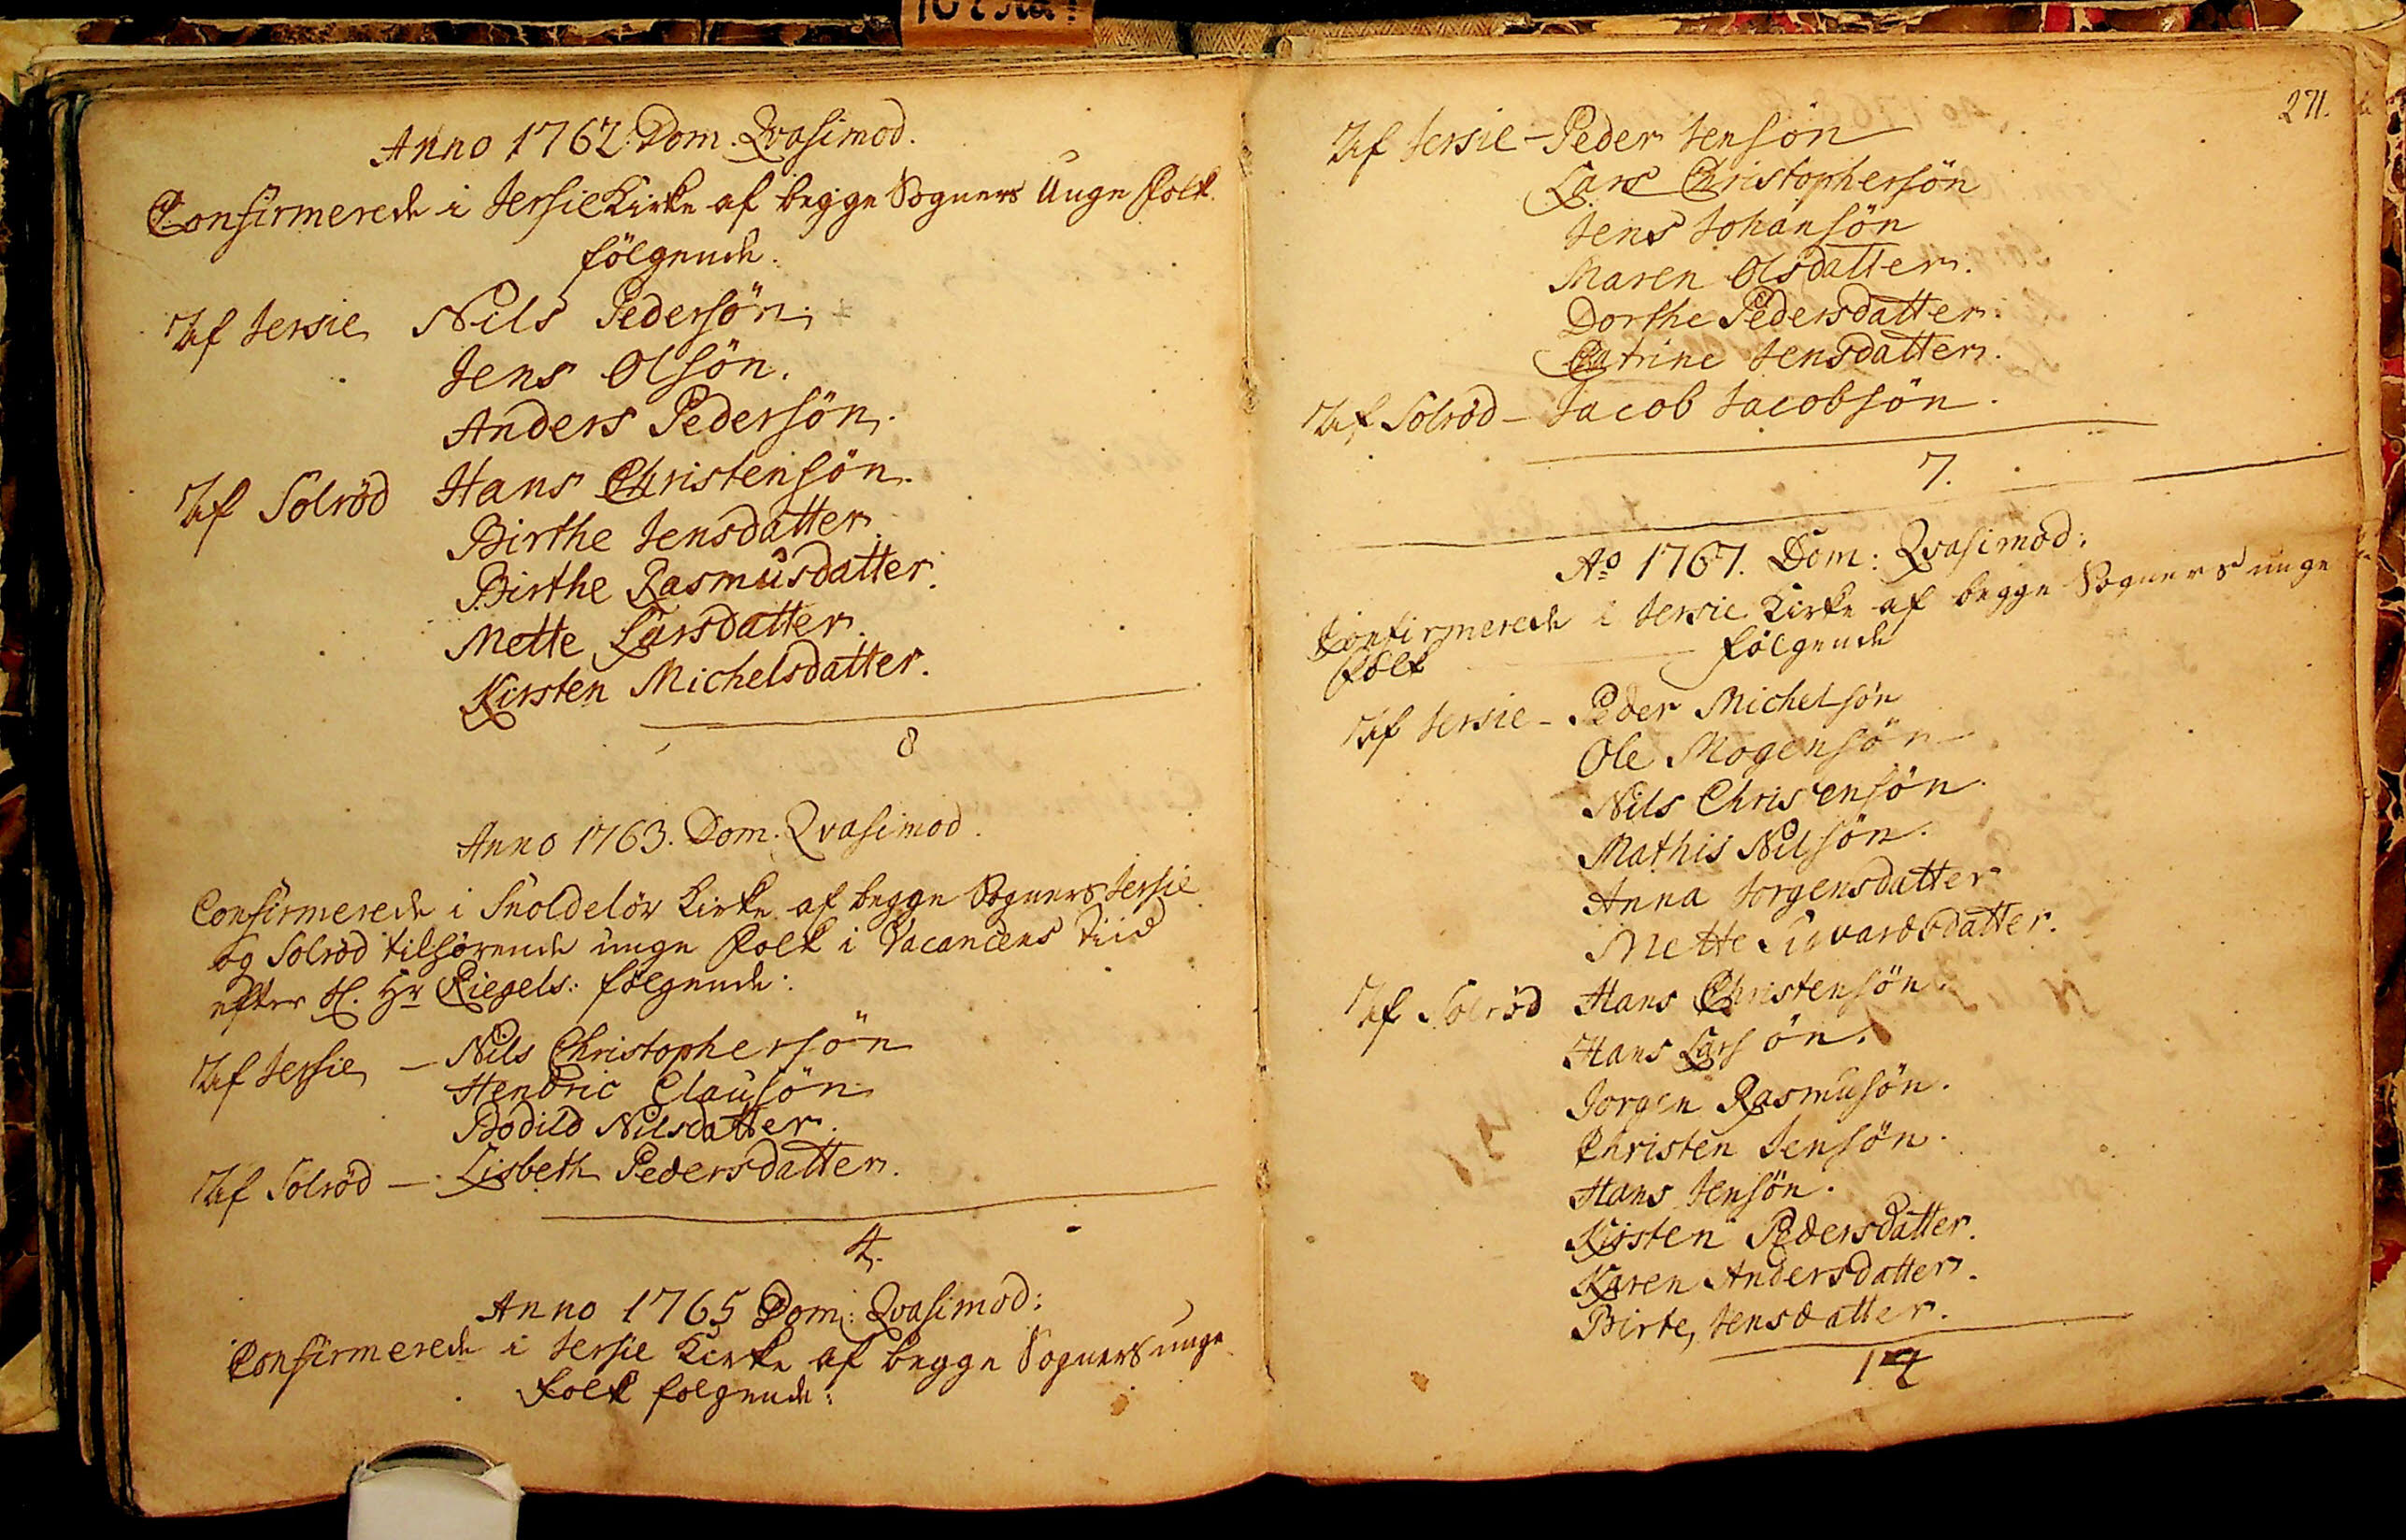Anno 1762. Dom. Qvasimod.
Confirmerede i Jersie Kirke af begge Sogners Unge Folk
følgende:
Af Jersie Nils Pedersøn.
Jens Olsøn.
Anders Pedersøn.
Af Solrød Hans Christensøn.
Birthe Jensdatter.
Birthe Rasmusdatter.
Mette Larsdatter.
Kirsten Michelsdatter.
8.
Anno 1763. Dom. Qvasimod.
Confirmerede i Snoldeløv Kirke af begge Sogners Jersie
og Solrød tilhørende unge Folk i Vacancens Tiid
efter Sl. Hr Riegels: følgende:
Af Jersie - Nils Christophersøn
Hendric Clausøn
Bodild Nilsdatter.
Af Solrød - Lisbeth Pedersdatter.
4.
Ano 1765 Dom: Qvasimod:
Confirmerede i Jersie Kirke af begge Sognes unge
folk følgende:
271.
Af Jersie - Peder Jensøn
Lars Christophersøn
Jens Johansøn
Maren Olsdatter.
Dorthe Pedersdatter.
Catrine Jensdatter.
Af Solrød - Jacob Jacobsøn.
7.
Ao 1767. Dom: Qvasimod:
Confirmerede i Jersie Kirke af begge Sogners unge
Folk
følgende
Af Jersie - Peder Michelsøn
Ole Mogensøn
Nils Christensøn
Mathis Nilsøn.
Anna Jørgensdatter
Mette Sigvardsdatter.
Af Solrød Hans Christensøn.
Hans Larsøn.
Jørgen Rasmusøn.
Christen Jensøn.
Hans Jensøn.
Kirsten Pedersdatter.
Karen Andersdatter.
Birte Jensdatter.
14
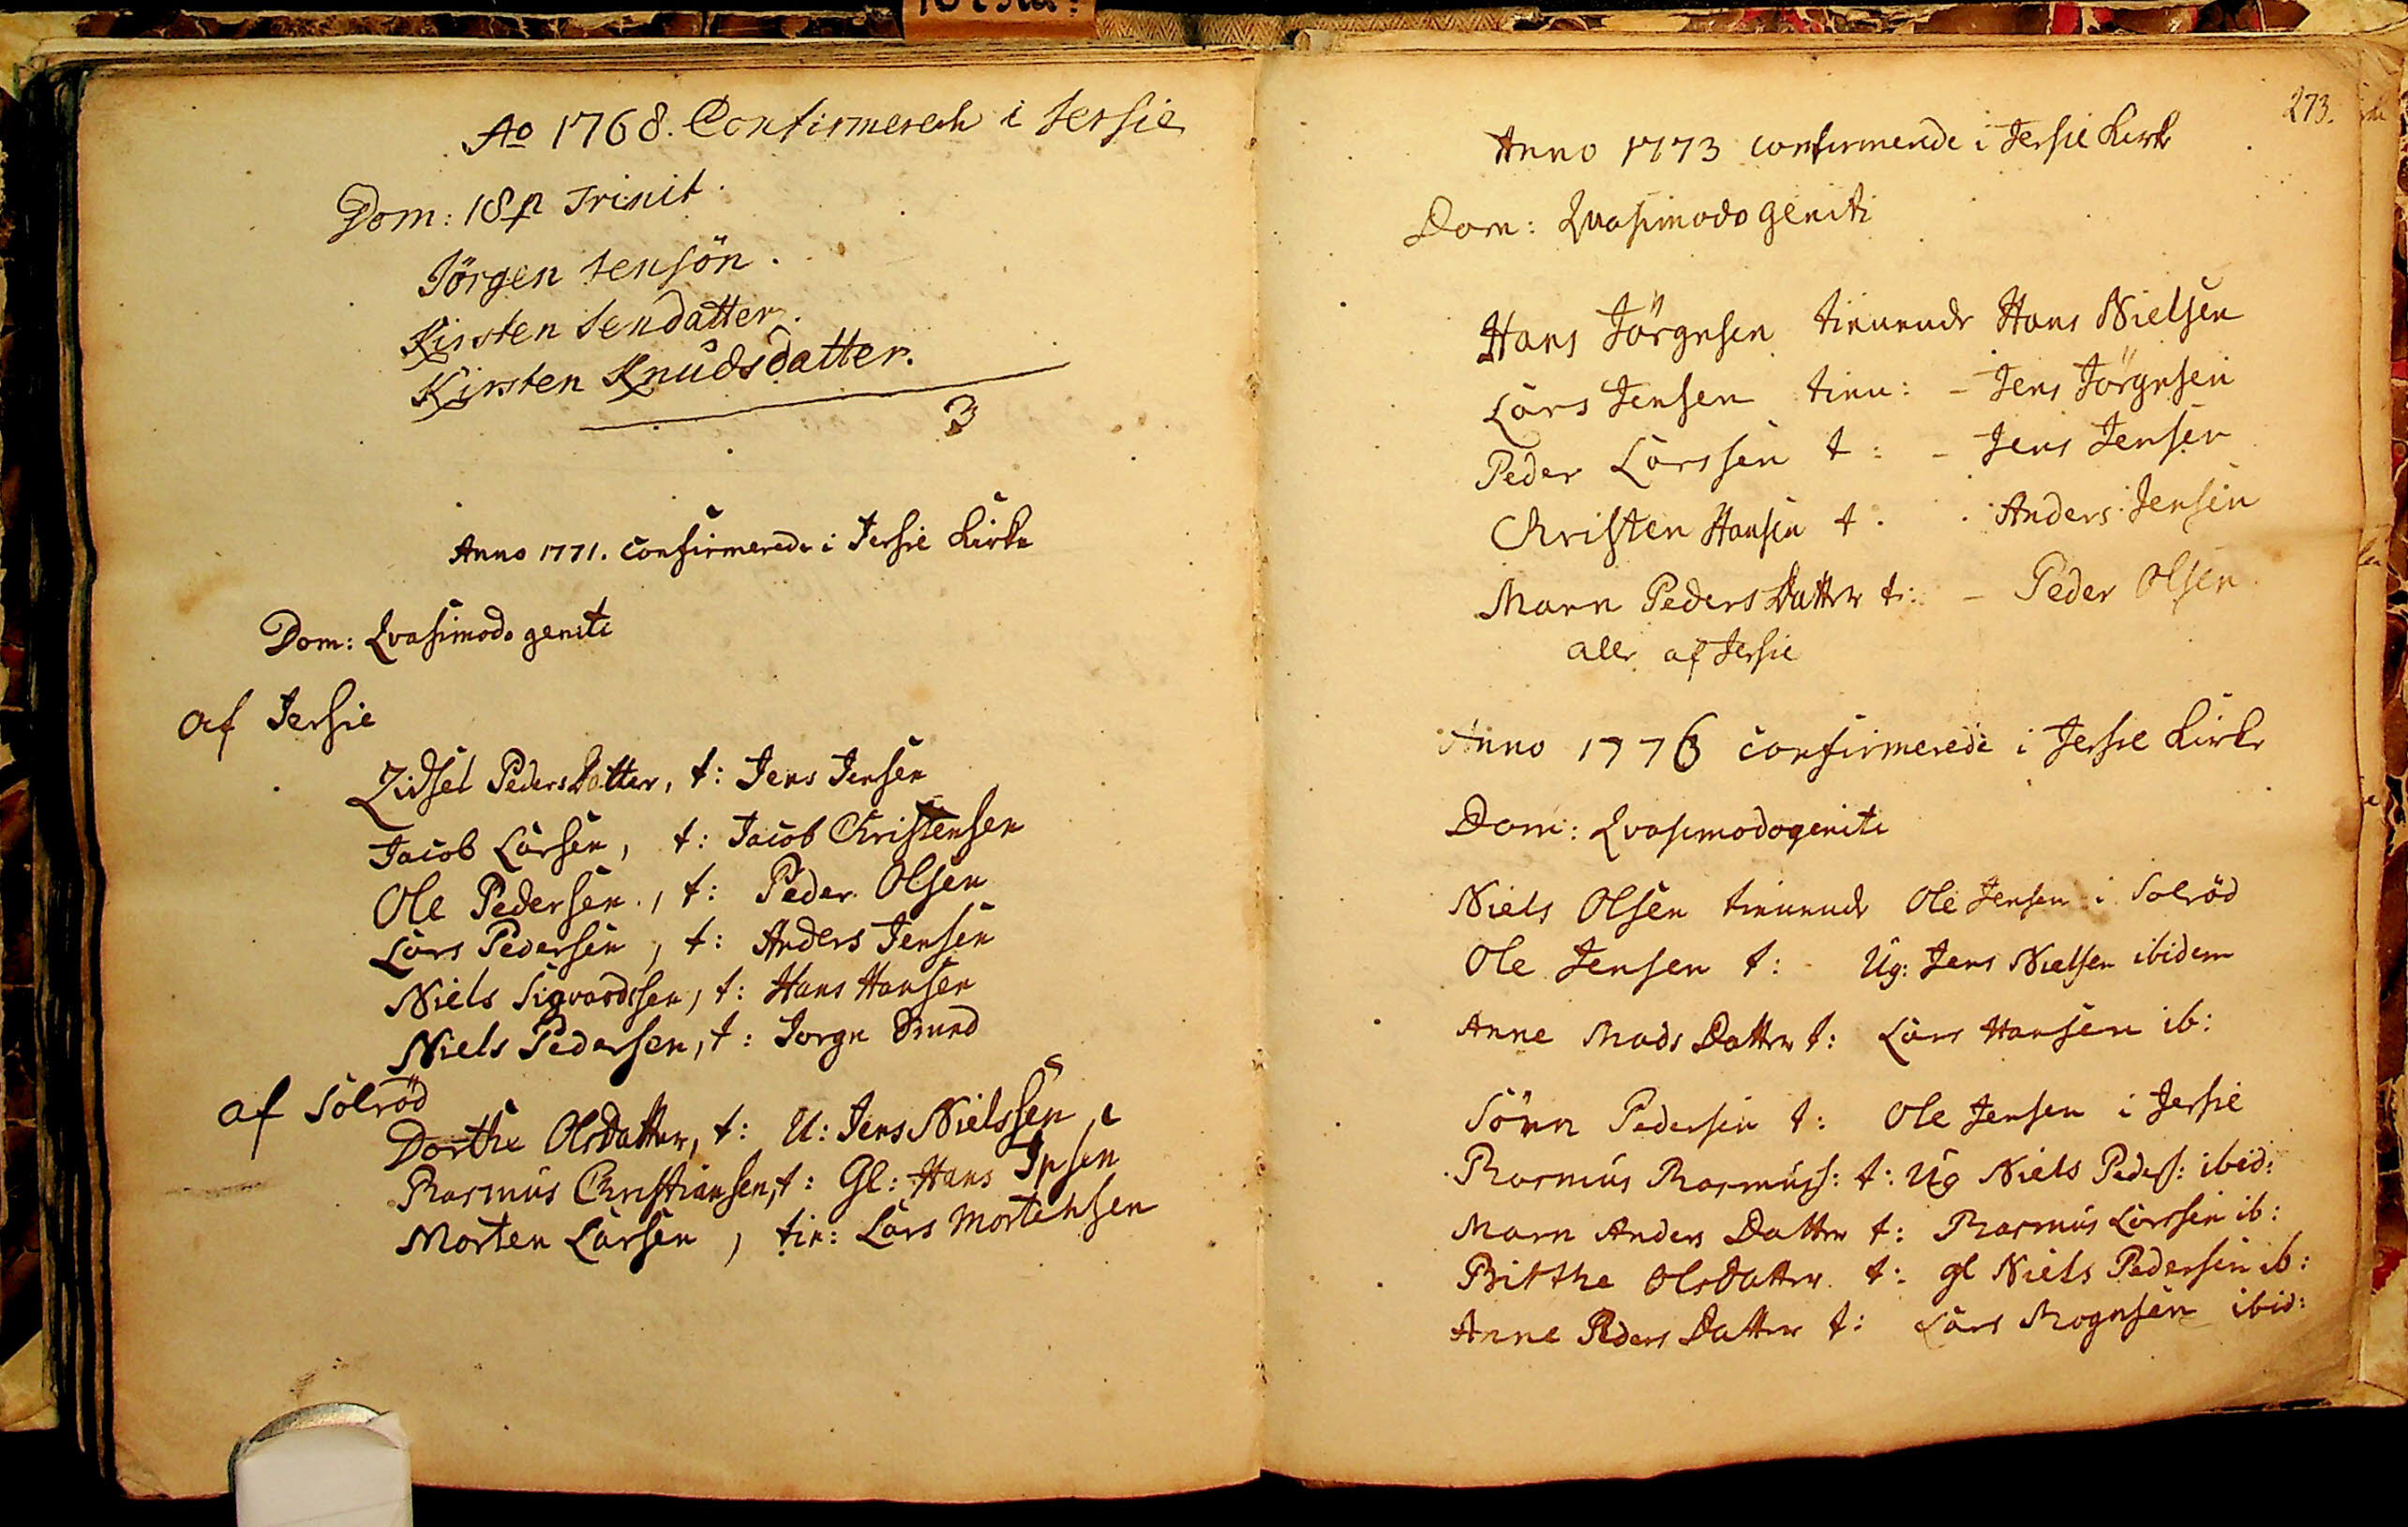Ao 1768. Confirmerede i Jersie
Dom: 18 p Trinit:
Jørgen Jensøn.
Kirsten Jensdatter.
Kirsten Knudsdatter.
3
Anno 1771. Confirmerede i Jersie Kirke
Dom: Qvasimodogeniti
af Jersie
Zidsel Peders Datter, t: Jens Jensen
Jacob Larsen, t: Jacob Christensen
Ole Pedersen, t: Peder Olsen
Lars Pedersen, t: Anders Jensen
Niels Sigvardssen, t: Hans Hansen
Niels Pedersen, t: Jørgn Smed
af Solrød
Dorthe OlsDatter, t: U: Jens Nielssen
Rasmus Christiansen, t: Gl: Hans Ipsen
Morten Larsen, tie: Lars Mortensen
273.
Anno 1773 Confirmerede i Jersie Kirke
Dom: Qvasimodo Geniti
Hans Jørgnsen tienende Hans Nielsen
Lars Jensen tien: - Jens Jørgnsen
Peder Larssen t: - Jens Jensen
Christen Hansen t: Anders Jensen
Marn Peders Datter t: - Peder Olsen.
Alle af Jersie
Anno 1776 confirmerede i Jersie Kirke
Dom: Qvasimodogeniti
Niels Olsen tienende Ole Jensen i Solrød
Ole Jensen t: Ug: Jens Nielsen ibidem
Anne Mads Datter t: Lars Hansen ib:
Sørn Pedersen t: Ole Jensen i Jersie
Rasmus Rasmuss: t: Ug Niels Peders: ibid:
Marn Anders Datter t: Rasmus Larssen ib:
Birthe Ols Datter t: gl Niels Pedersen ib:
Anne Peders Datter t: Lars Mognsen ibid: